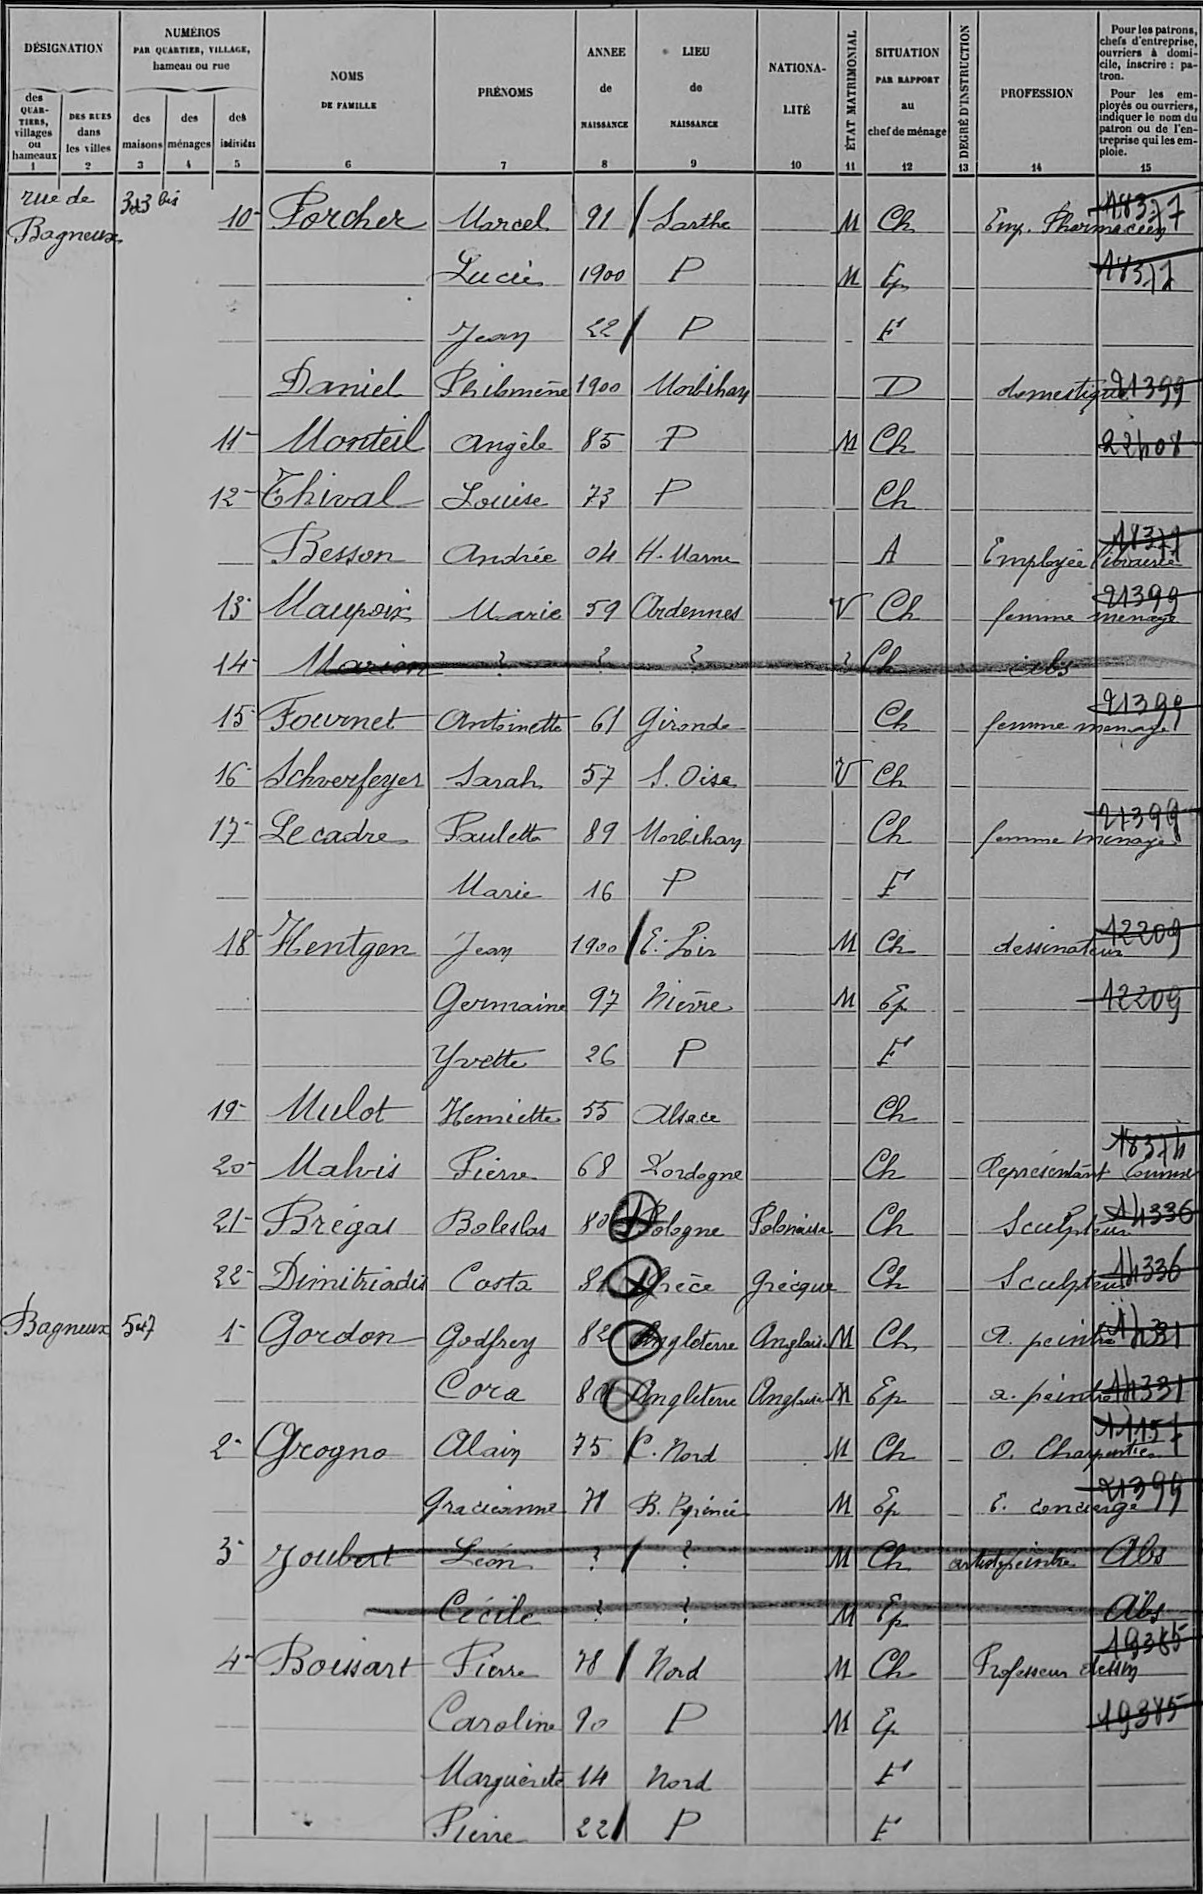Porcher/Marcel/91/Sarthe/¤/M/Ch/¤/Emp Pharmacien/?18377
¤/Lucie/1900/P/¤/M/Ep/¤/¤/18377
¤/Jean/22/P/¤/¤/F/¤/¤/¤
Daniel/Philomène/1900/Morbihan/¤/¤/D/¤/domestique/21399
Monteil/Angèle/85/P/¤/M/Ch/¤/¤/22408
Thival/Louise/73/P/¤/¤/Ch/¤/¤/¤
Besson/Andrée/03/H Marne/¤/¤/A/¤/Employée libraie/!18379
Maupoix/Marie/59/ardennes/¤/V/Ch/¤/femme ménage/?21399
Marion/¤/¤/¤/¤/¤/Ch/¤/abs/¤
Fournet/Antoinette/61/Gironde/¤/¤/Ch/¤/femme méange/?21399
Schverfeyer/Sarah/57/S Oise/¤/V/Ch/¤/¤/¤
Le cadre/Paulette/89/Morbihan/¤/¤/Ch/¤/femme méange/?21399
¤/Marie/16/P/¤/¤/F/¤/¤/¤
Hentgen/Jean/1900/E Loir/¤/M/Ch/¤/dessinateur/?12209
¤/Germaine/97/Nièvre/¤/M/Ep/¤/¤/12209
¤/Yvette/26/P/¤/¤/F/¤/¤/¤
Mulot/Henriette/55/Alsace/¤/¤/Ch/¤/¤/¤
Malvis/Pierre/68/Dordogne/¤/¤/Ch/¤/Représentant comm/?18374
Brégas/Boleslas/80/Pologne/Polonaise/¤/Ch/¤/Sculpteur/?14336
Dimitriadis/Costa/81/Grève/Grecque/¤/Ch/¤/Sculpteur/?14336
Gordon/Godfrey/82/Angleterre/Anglais/M/Ch/¤/A peintre/14331
¤/Cora/80/Angleterre/Anglaise/M/Ep/¤/a peintre/14331
Grogno/Alain/75/C Nord/¤/M/Ch/¤/O Charpentier/11157
¤/Gracienne/78/B Pyrénées/¤/M/Ep/¤/E concierge/?21399
Joubert/Léon/¤/¤/¤/M/Ch/¤/artiste peintre/Abs
¤/Cécile/¤/¤/¤/M/Ep/¤/¤/Abs
Boissart/Pierre/78/Nord/¤/M/Ch/¤/Professeur dessin/?19385
¤/Caroline/90/P/¤/M/Ep/¤/¤/19385
¤/Marguerite/14/Nord/¤/¤/F/¤/¤/¤
¤/PIerre/22/P/¤/¤/F/¤/¤/¤
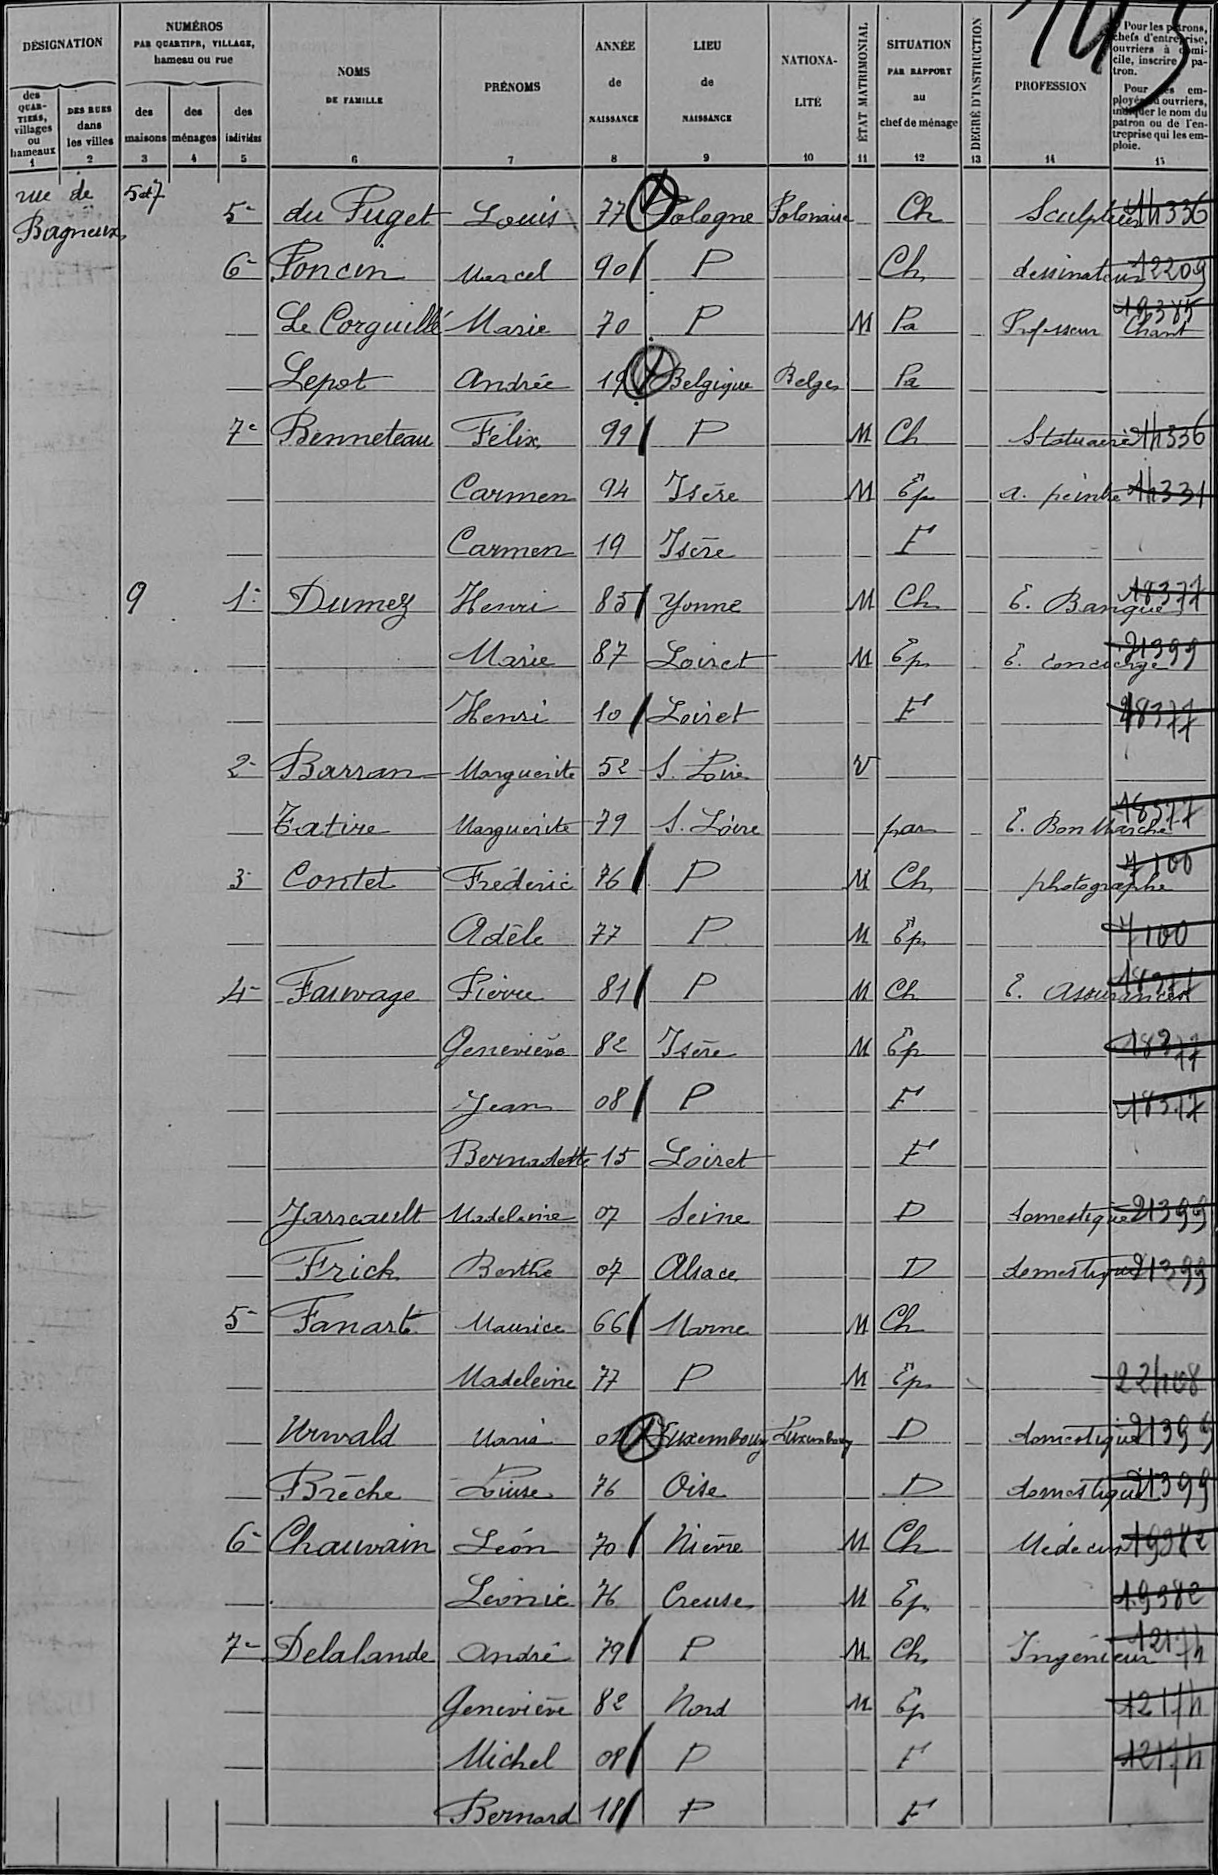du Puget/Louis/77/Pologne/Polonaise/¤/Ch/¤/Sculpteur/14336
Poncin/Marcel/90/P/¤/¤/Ch/¤/dessinateur/12209
Le Corguillé/Marie/70/P/¤/M/Pa/¤/Professeur Chant/?19385
Lepot/Andrée/19/Belgique/Belge/¤/Pa/¤/¤/¤
Benneteau/Félix/99/P/¤/M/Ch/¤/Statuaire/14336
¤/Carmen/94/ISère/¤/M/Ep/¤/a peintre/14331
¤/Carmen/19/Isère/¤/¤/F/¤/¤/¤
Dumez/Henri/85/Yonne/¤/M/Ch/¤/E Banque/18377
¤/MArie/87/Loiret/¤/M/Ep/¤/E concierge/21399
¤/Henri/10/Loiret/¤/¤/F/¤/¤/18377
BArran/Marguerite/52/S Loire/¤/V/¤/¤/¤/¤
Tatire/Marguerite/79/S Loire/¤/¤/par/¤/E Bon Marché/?18377
Contel/Frédéric/76/P/¤/M/Ch/¤/photographe/7100
¤/Adèle/77/P/¤/M/Ep/¤/¤/7100
Fauvage/Pierre/81/P/¤/M/Ch/¤/E assurances/18377
¤/Geneviève/82/Isère/¤/M/Ep/¤/¤/18377
¤/Jean/08/P/¤/¤/F/¤/¤/18377
¤/Bernadette/15/Loiret/¤/¤/F/¤/¤/¤
JArreault/Madeleine/07/Seine/¤/¤/D/¤/domestique/21399
Frick/Berthe/07/Alsace/¤/¤/D/¤/domestique/21399
Fanart/Maurice/66/Marne/¤/M/Ch/¤/¤/¤
¤/Madeleine/77/P/¤/M/Ep/¤/¤/22408
Urwald/Maria/04/Luxembourg/Luxembourg/¤/D/¤/domestique/21399
Brèche/Louise/76/Oise/¤/¤/D/¤/domestique/21399
Chauvain/Léon/70/Nièvre/¤/M/Ch/¤/Médecin/19382
¤/Léonie/76/Creuse/¤/M/Ep/¤/¤/19382
Delalande/André/79/P/¤/M/Ch /¤/Ingénieur/12174
¤/Geneviève/82/Nord/¤/M/Ep/¤/¤/12174
¤/Michel/08/P/¤/¤/F/¤/¤/12174
¤/Bernard/18/P/¤/¤/F/¤/¤/¤